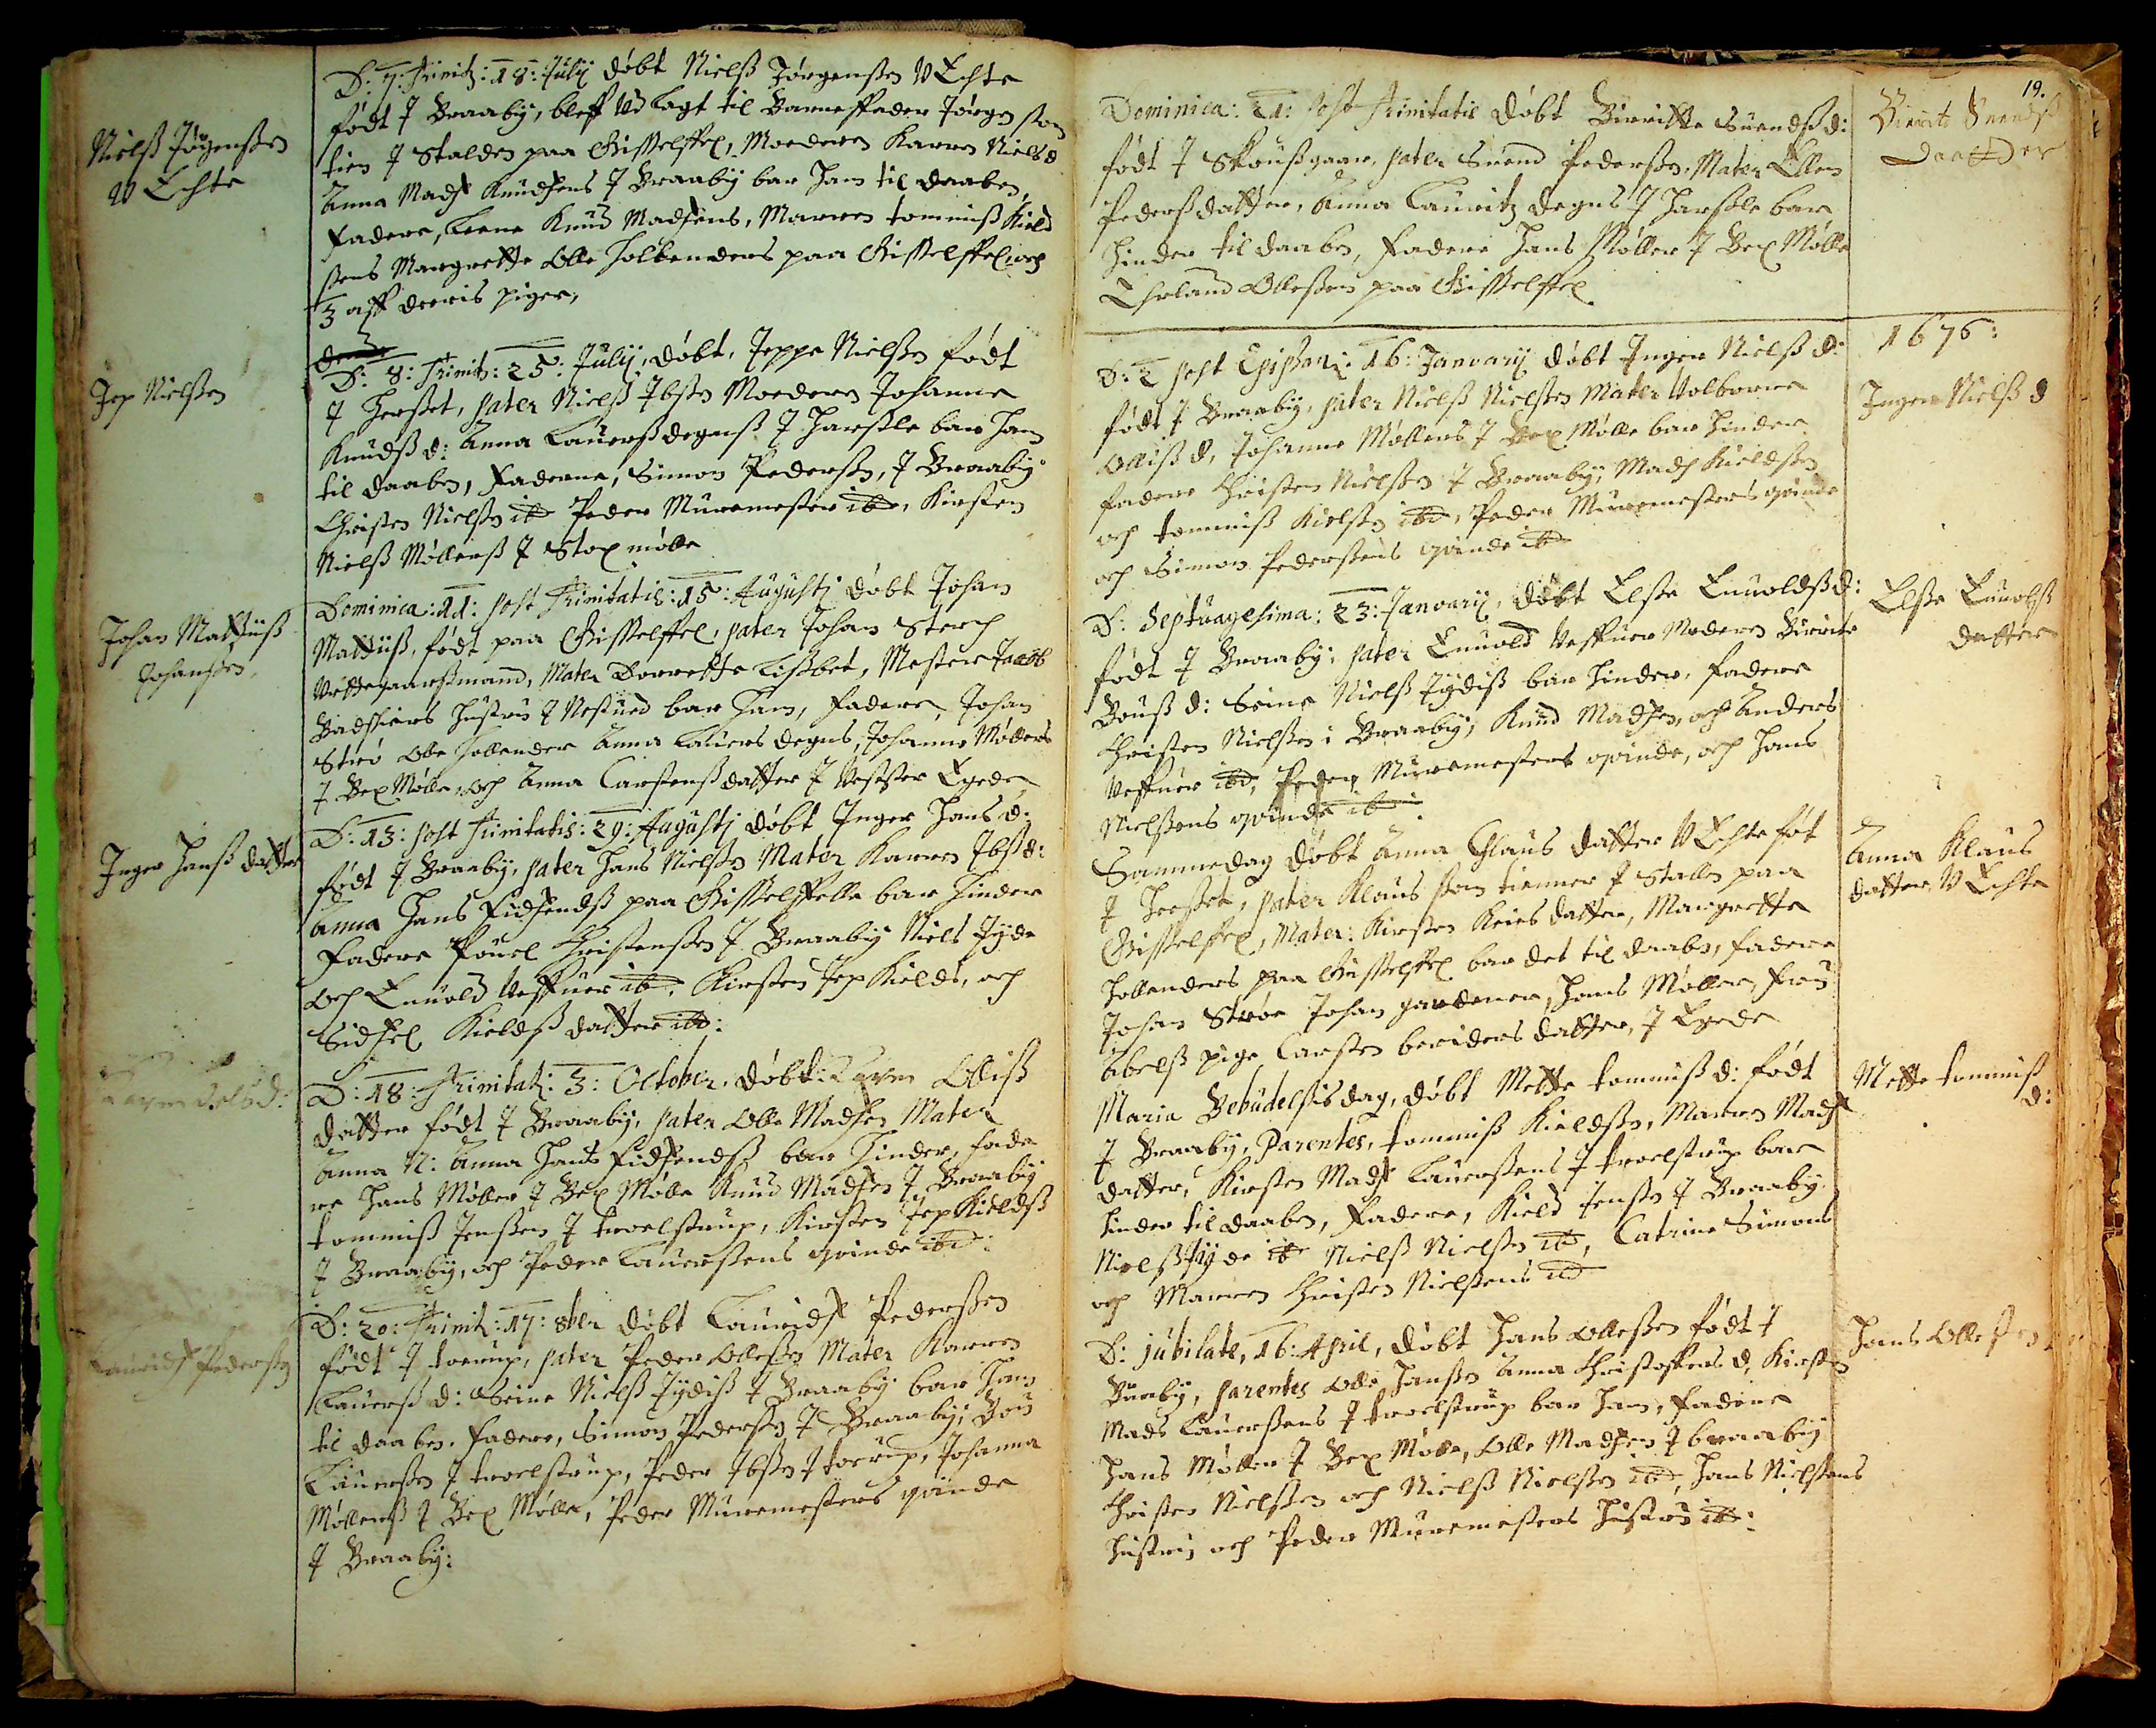Niels Jørgensen 
U Echte 
D: 7: Trinitz: 18: July døbt Niels Jørgensen U Echte 
født J Braaby, bleff Udlagt til BarnefFader Jørgen  som
tien J Stalden paa Gisselffel, Moderen Karren Niels d.
Anna Mads Knudsens J Braaby bar ham til daaben.
Fadere, Leene Knud Madsens, Marren Tommis Kield
sens Margrette Olle Hollenders paa Gisselffel och
3 aff deeris piger.
Jeppe Nielsen 
D: 8: Trinitz: 25: July, døbt, Jeppe Nielsen født
J Herset. Pater Niels Jbsen. Moderen Johanne
Knuds d: Anna Lauers Degns J Harsle bar ham
til daaben, Faderne, Simon Pedersen, J Braaby
Christen Nielsen ibd. Peder Muremester ibd. Kirsten
Niels Møllers J Stoxmølle.
Johan Mattiis 
Johansen 
Dominica: 11: post Trinitatis: 15: Augustj døbt Johan
Mattiis, født paa Gisselffel. Pater Johan Sterch
Urttegaarsmand, Mater Dorrette Lisbet, Mester Jacob
Badskirs Hustru J Nestued bar ham, Fadere, Johan
Strø. Olle hollender Anna Lauers Degns, Johanne Møllers
J Bex Mølle, och Anna Carstens datter J Vester Egede.
Jnger Hans datter 
D: 13: post Trinitatis: 29: Augustj døbt Jnger Hans d: 
født J Braaby, Pater Hans Nielsen Mater Karren Jbs d:
Anna Hans Fidsends paa Gisselffelde bar hinder.
Fadere Pouel Christensen J Braaby Niels Jyde
Och Enuold Veffuer ibd. Kirsten Jep Kields, och
Sidsel Kields datter ibd.
Karen## Oels d:
D: 18: Trinitat##: 3: October døbt Karen## Ollis
datter født J Braaby, Pater Olle Madsen Mater
Anna N: Anna Hans Fidsends bar hinder. Fade
re Hans Møller J Bex Mølle. Knud Madsen J Braaby
Tommis Jensen J Troelstrup, Kirsten Jep Kields
J Braaby, och Peder Lauersens qvinde ibd.
Lauridz Pedersen 
D: 20: Trinit: 17: 8ber døbt Laurids Pedersen
født J Toerup. Pater Peder Ollesen Mater Karren
Lauers d: Seine Niels Jydis J Braaby bar ham
til daaben. Fadere, Simon Pedersen J Braaby. Bou
Lauersen J Troelstrup. Peder Jbsen J Toerup, Johanna
Møllers J Bex Mølle, Peder Murermesters qvinde
J Braaby:
Dominica: 21: post Trinitatis døbt Birritte Suends d:
født J Skousgaar. Pater Suend Pedersen. Mater Ellen
Pedersdatter, Anna Lauritz Degns J Harsle bar
hinder til daaben, Fadere Hans Møller J Bex Mølle
Ehrland Ollesen paa Gisselffel.
19.
Birrite## Suends
Daatter
D: 2 post Epiphan: 16: Janoary døbt Jnger Niels d:
født J Braaby, Pater Niels Nielsen. Mater Valborre
Ollis d. Johanne Møllers J Bex Mølle bar hinder
Fadere Christen Nielsen J Braaby; Mads Kieldsen
och Tommis Kielsen ibd. Peder Muremesters qvinde
och Simon Pedersens qvinde ibd.
1676:
Jnger Niels d:
D: Septuagesima: 23: january døbt Else Enuolds d: 
født J Braaby; Pater Enuold Veffuer. Moderen Birrite
Bous d: Seine Niels Jydis bar hinder, Fadere
Christen Nielsen i Braaby. Knud Madsen, och Anders
Veffuer ibd. Peder Muremesters qvinde, och Hans
Nielsens qvinde ibd.
Else Enuolds 
datter
Samme dag døbt Anna Claus datter U Echte føt 
J Heeset, Pater Klaus som tiener J Stallen paa
Gisselfel, Mater Kirsten Keies datter, Margrette
Hollenders paa Gisselfel bar det til daaben, Fadere
Johan Strøe, Johan gardener, Hans Møller, fru
Abels pige, Carsten beriders datter J Egede.
Anna Klaus 
datter U Echte
Maria Bebudelsesdag døbt Mette Tommis d: født 
J Braaby, Parentes, Tommis Kieldsen, Marren Mads
datter. Kirsten Mads Lauersens J Troelstrup bar
hinder til daaben, Fadere Kield Jensen J Braaby.
Niels Jyde ibd. Niels Nielsen ibd. Catrine Simons
och Marren Christen Nielsens ibd.
Mette Tommis
d:
D: jubilate, 16: April, døbt Hans Ollesen født J 
Braaby, Parentes: Olle Hansen. Anna Christoffers d. Kirsten
Mads Lauersens J Troelstrup bar ham, Fadere 
Hans Møller J Bex Mølle, Olle Madsen J Braaby.
Christen Nielsen och Niels Nielsen ibd. Hans Nielsens
Hustru; och Peder Muremesters Hustru ibd.
Hans Ollesen
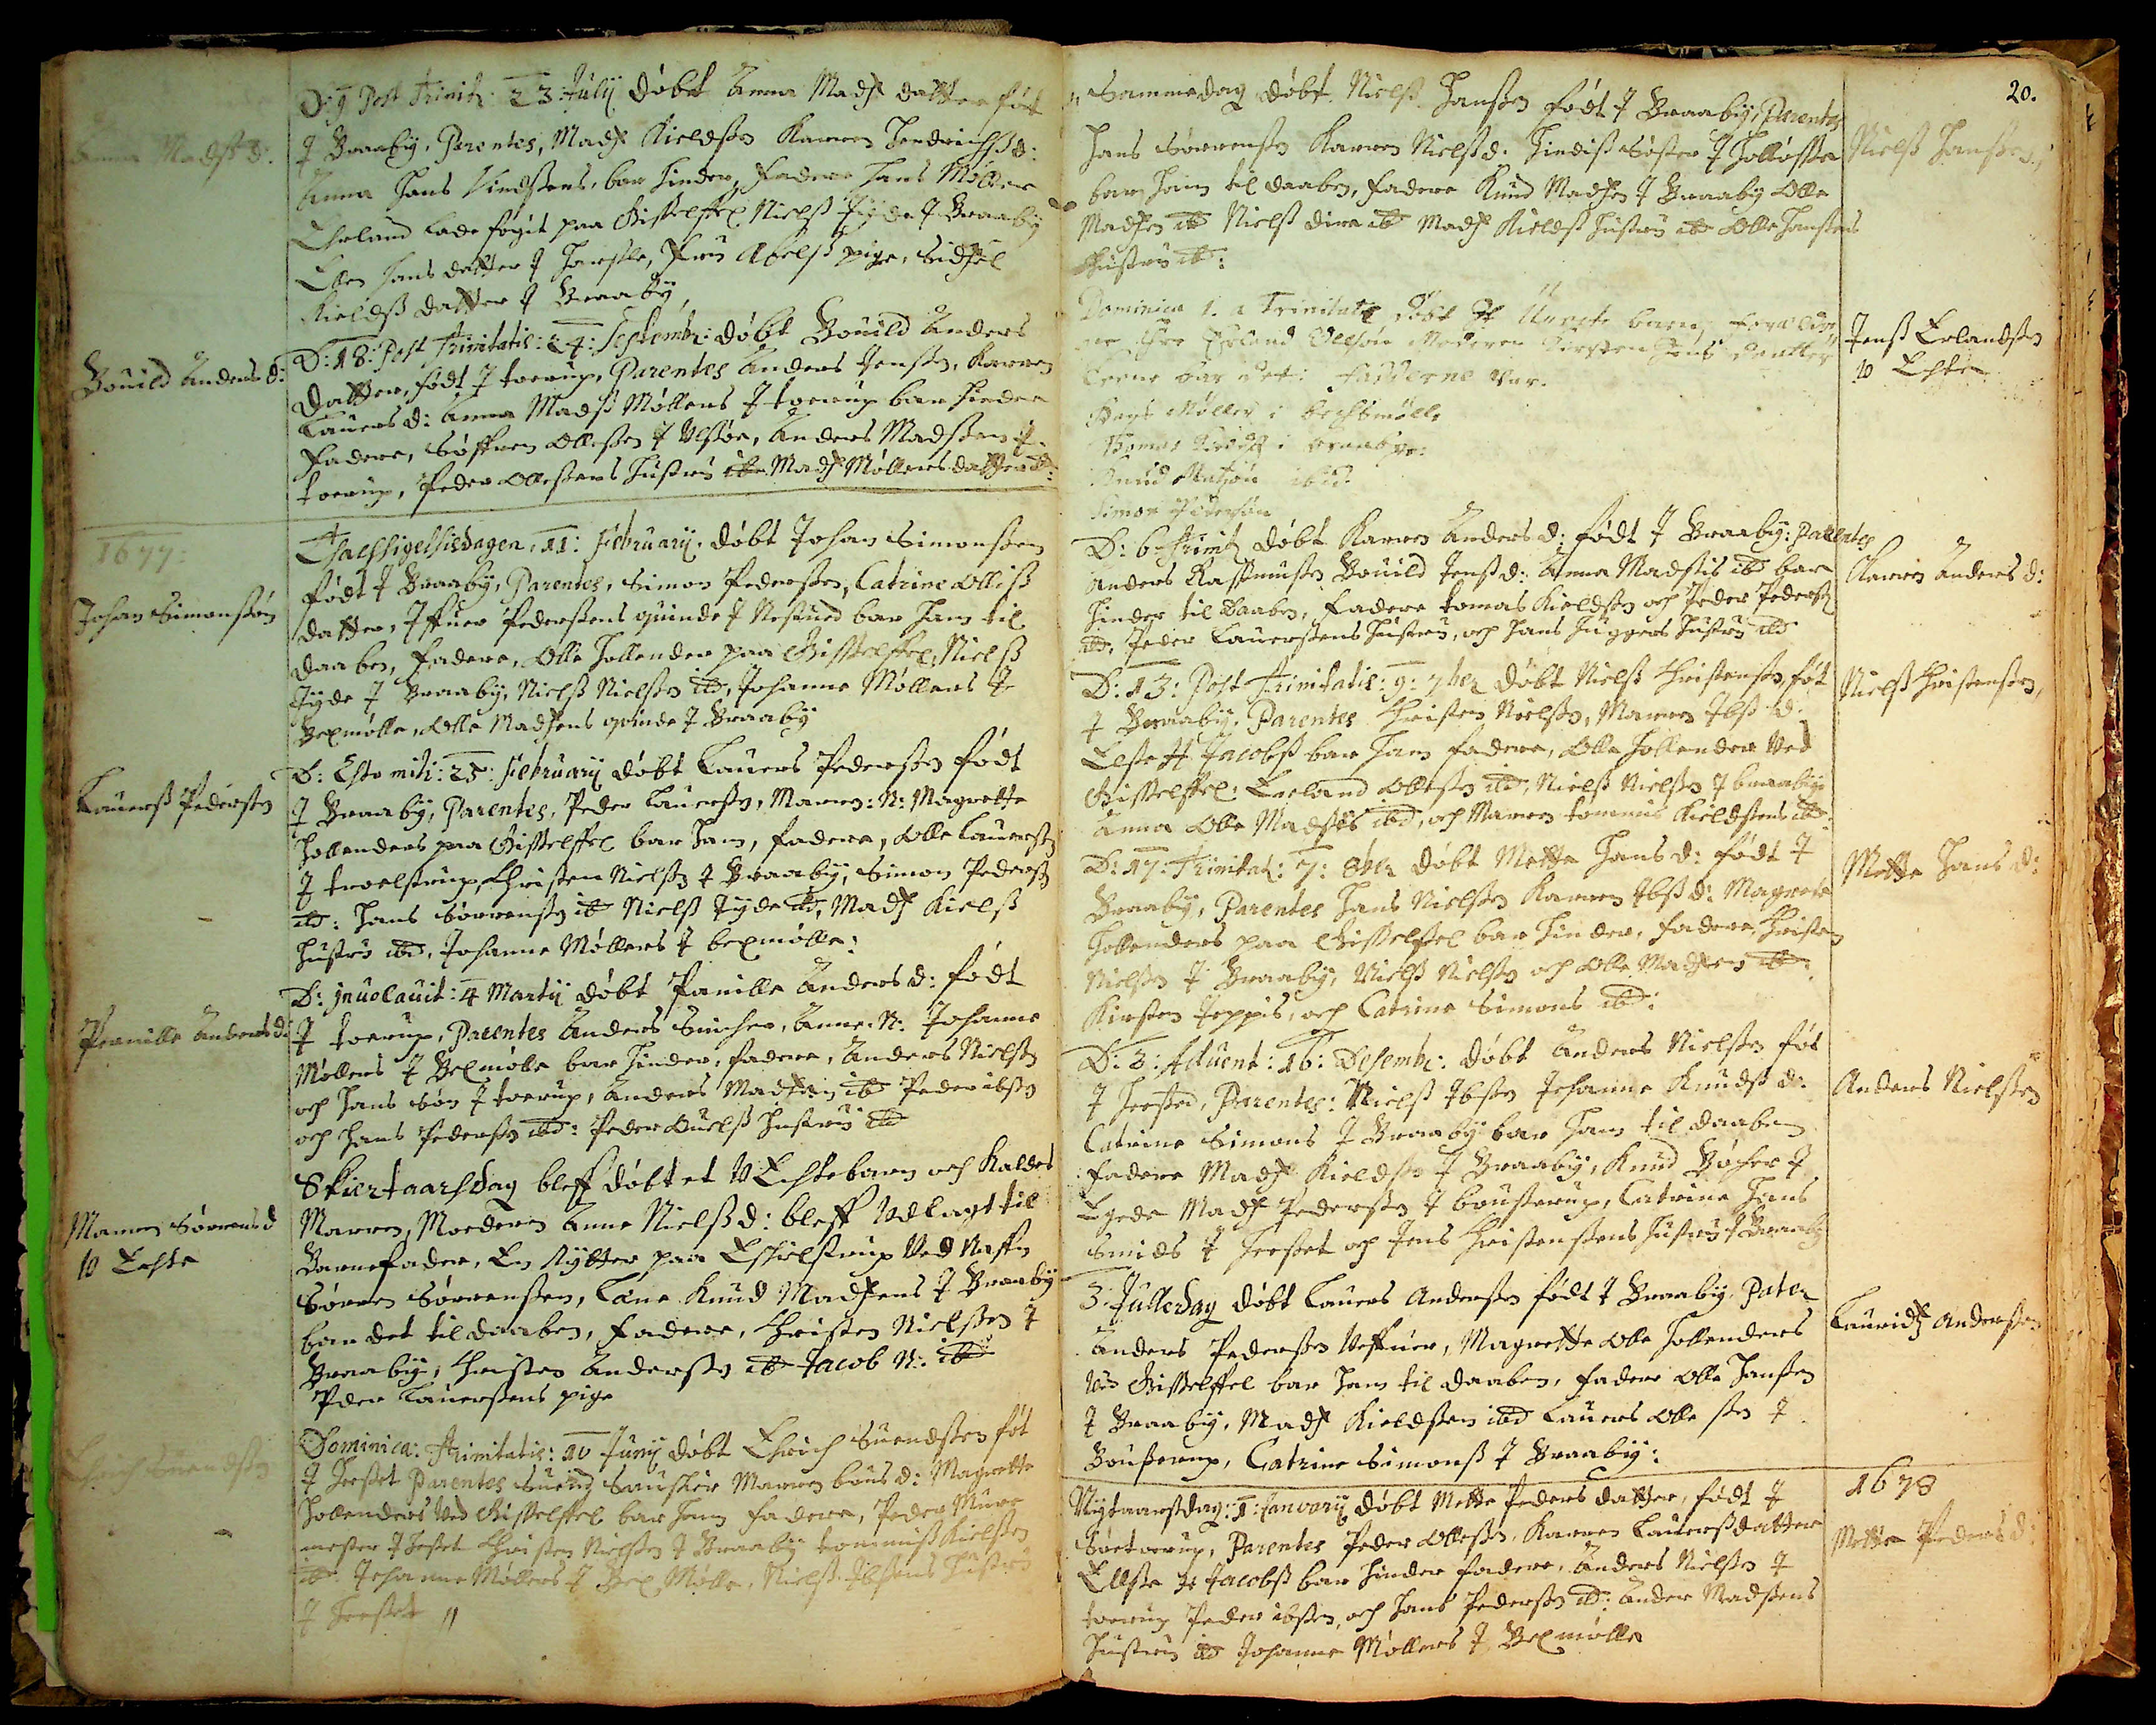Anna Mads D: 
D: 9 Post Trinitz: 23: July døbt Anna Mads datter født
J Braaby, Parentes, Mads Kieldsen, Karren Hendrichsen d:
Anna Hans Vindsens, bar hinder, Fadere Hans Møller
Ehrland Lade fogit paa Gisselfel. Niels Jyde J Braaby.
Ellen Hans datter J Harsle, Fru Abels pige, Sidsel
Kields datter J Braaby.
Bouild Anders d: 
D:18: Post Trinitatis: 24: Septembr: døbt Bouild Anders
datter, født J Toerup, Parentes: Anders Jensen, Karren
Lauers d: Anna Mads Møllers J Toerup bar hinder.
Fadere, Søffren Ollesen J Ulsøe. Anders Madsen J
Toerup, Peder Ollesens Hustru ibd. Madz Møllers datters.##
1677:
Johan Simonsøn
Thachsigelsisdagen 11: February. døbt Johan Simonsøn
født J Braaby, Parentes, Simon Pedersen, Catrine Ollis
datter, Jffuer Pedersens qvinde J Nestued bar ham til
daaben, Fadere, Olle Hollender paa Gisselffel, Niels
Jyde J Braaby, Niels Nielsen ibd. Johanne Møllers J
Bexmølle, Olle Madsens qvinde J Braaby.
Lauers Pedersen 
D: Esto Mihi: 25: February døbt Lauers Pedersen født 
J Braaby, Parentes, Peder Lauersen, Marren: N: Magrette
Hollenders paa Gisselffel bar ham, Fadere, Olle Lauersen
J Troelstrup, Christen Nielsen J Braaby, Simon Pedersen
ibd. Hans Sørrensen ibd. Niels Jyde ibd. Mads Kields
Hustru ibd. Johanne Møllers J Bexmølle:
Panille Andersd:
D: jnuocauit##: 4: Marty døbt Panille Anders d: født
J Toerup, Parentes Anders Snicher, Anne. N.: Johanne
Møllers J Bexmølle bar hinder, Fadere, Anders Nielsen
och Hans Søn J Toerup, Anders Madsen ibd. Peder Ibsen
och Hans Pedersen ibd. Peder Ouels Hustru ibd.
Marren Sørrens d. 
U Echte
Skiertaarsdag bleff døbt et U Echte barn och kaldes 
Marren, Moederen Anne Niels d: bleff Udlagt til
Barnefader, En Rytter paa Eskielstrup Ved Naffn
Sørren Sørrensen, Læne Knud Madsens J Braaby
bar det til daaben, Fadere, Christen Nielsen J
Braaby, Christen Andersen ibd. Jacob N: ibd.
Peder Lauersens pige.
Ehrich Suendsen 
Dominica: Trinitatis: 10 Juny døbt Ehrich Suendsen føt
J Heeset. Parentes: Suend Sauskir. Marren Bous d: Magrette
Hollenders ved Gisselffel bar ham. Fadere, Peder Mure
mester J Heset, Christen Nielsen J Braaby, Tommis Kielsen
ibd. Johanne Møllers J Bex Mølle, Niels Jbsens Hustru
J Heeset.
Samme dag døbt Niels Hansen født J Braaby, Parentes 
Hans Sørrensen Karren Niels d. Hindis Søster J Holløsse##
bar ham til daaben, Fadere Knud Madsen J Braaby, Olle
Madsen ibd. Niels Dire ibd. Mads Kields Hustru ibd. Olle Hansens
Hustru ibd:
20.
Niels Hansen
Dominica 1. a Trinitatis døbt Et U Echte barn, Forældre##
##Ehre Erland Ollsøn. Moderen Kirsten Jens datter
Leene bar det: Fadderne var.
Hans Møller i Bechsmølle
Thomas Knuds i Braabye.
Knud Matzøn ibid.
Simon Pedersøn. 
Jens Erlandsen 
U Echte
D: 6 Trinit døbt Karren Anders d. født J Braaby: Parentes 
Anders Rassmusen Bouild Jens d: Anne Madsis ibd bar
hinder til Daaben, Fadere, Tomas Kieldsen och Peder Pedersen
ibd. Peder Lauersens Hustru, och Hans Huggers Hustru ibd.
Karren Anders d:
D: 13: Post Trinitatis: 9: 7ber døbt Niels Christensen føt 
J Braaby. Parentes Christen Nielsen, Marren Jbs d.
Else H Jacobs bar ham Fadere, Olle Hollender ved
Gisselfel. Erland Ollesen ibd. Niels Nielsen J Braaby
Anna Olle Madsis ibd. och Marren Tommis Kieldsens ibd:
Niels Christensen
D: 17: Trinitat: 7: 8ber døbt Mette Hans d: født J 
Braaby, Parentes Hans Nielsen Karren Jbs d: Magrete
Hollenders paa Gisselfel bar hinder, Fadere, Christen
Nielsen J Braaby, Niels Nielsen och Olle Madsen ibd.
Kirsten Jeppis och Catrine Simons ibd.
Mette Hans d:
D: 3: Aduent: 16: Desembr: døbt Anders Nielsen føt 
J Heesed, Parentes: Niels Jbsen Johanne Knuds d.
Catrine Simons J Braaby bar ham til daaben
Fadere Mads Kieldsen J Braaby, Knud Bøcher J
 Egede, Mads Pedersen i Bouserup, Catrine Hans
Smids J Heeset och Jens Christensens Hustru J Braaby.
Anders Nielsen
3. Julledag døbt Lauers Andersen født J Braaby. Pater: 
Anders Pedersen Veffuer, Magrette Olle Hollenders 
ved Gisselffel bar ham til daaben, Fadere Olle Hansen
J Braaby, Mads Kieldsen ibd. Lauers Ollesen J
Bouserup, Catrine Simons J Braaby:
Laurids Andersen
Nytaarsdag: 1: January døbt Mette Peders datter, født J 
Søetoerup, Parentes Peder Ollesen, Karren Lauers datter
Ellse H Jacobs bar hinder Fadere, Anders Nielsen J 
Toerup, Peder Ibsen, och Hans Pedersen ibd. Ander Madsens
Hustru ibd. Johanne Møllers J Bexmølle.
1678
Mette Peders d: